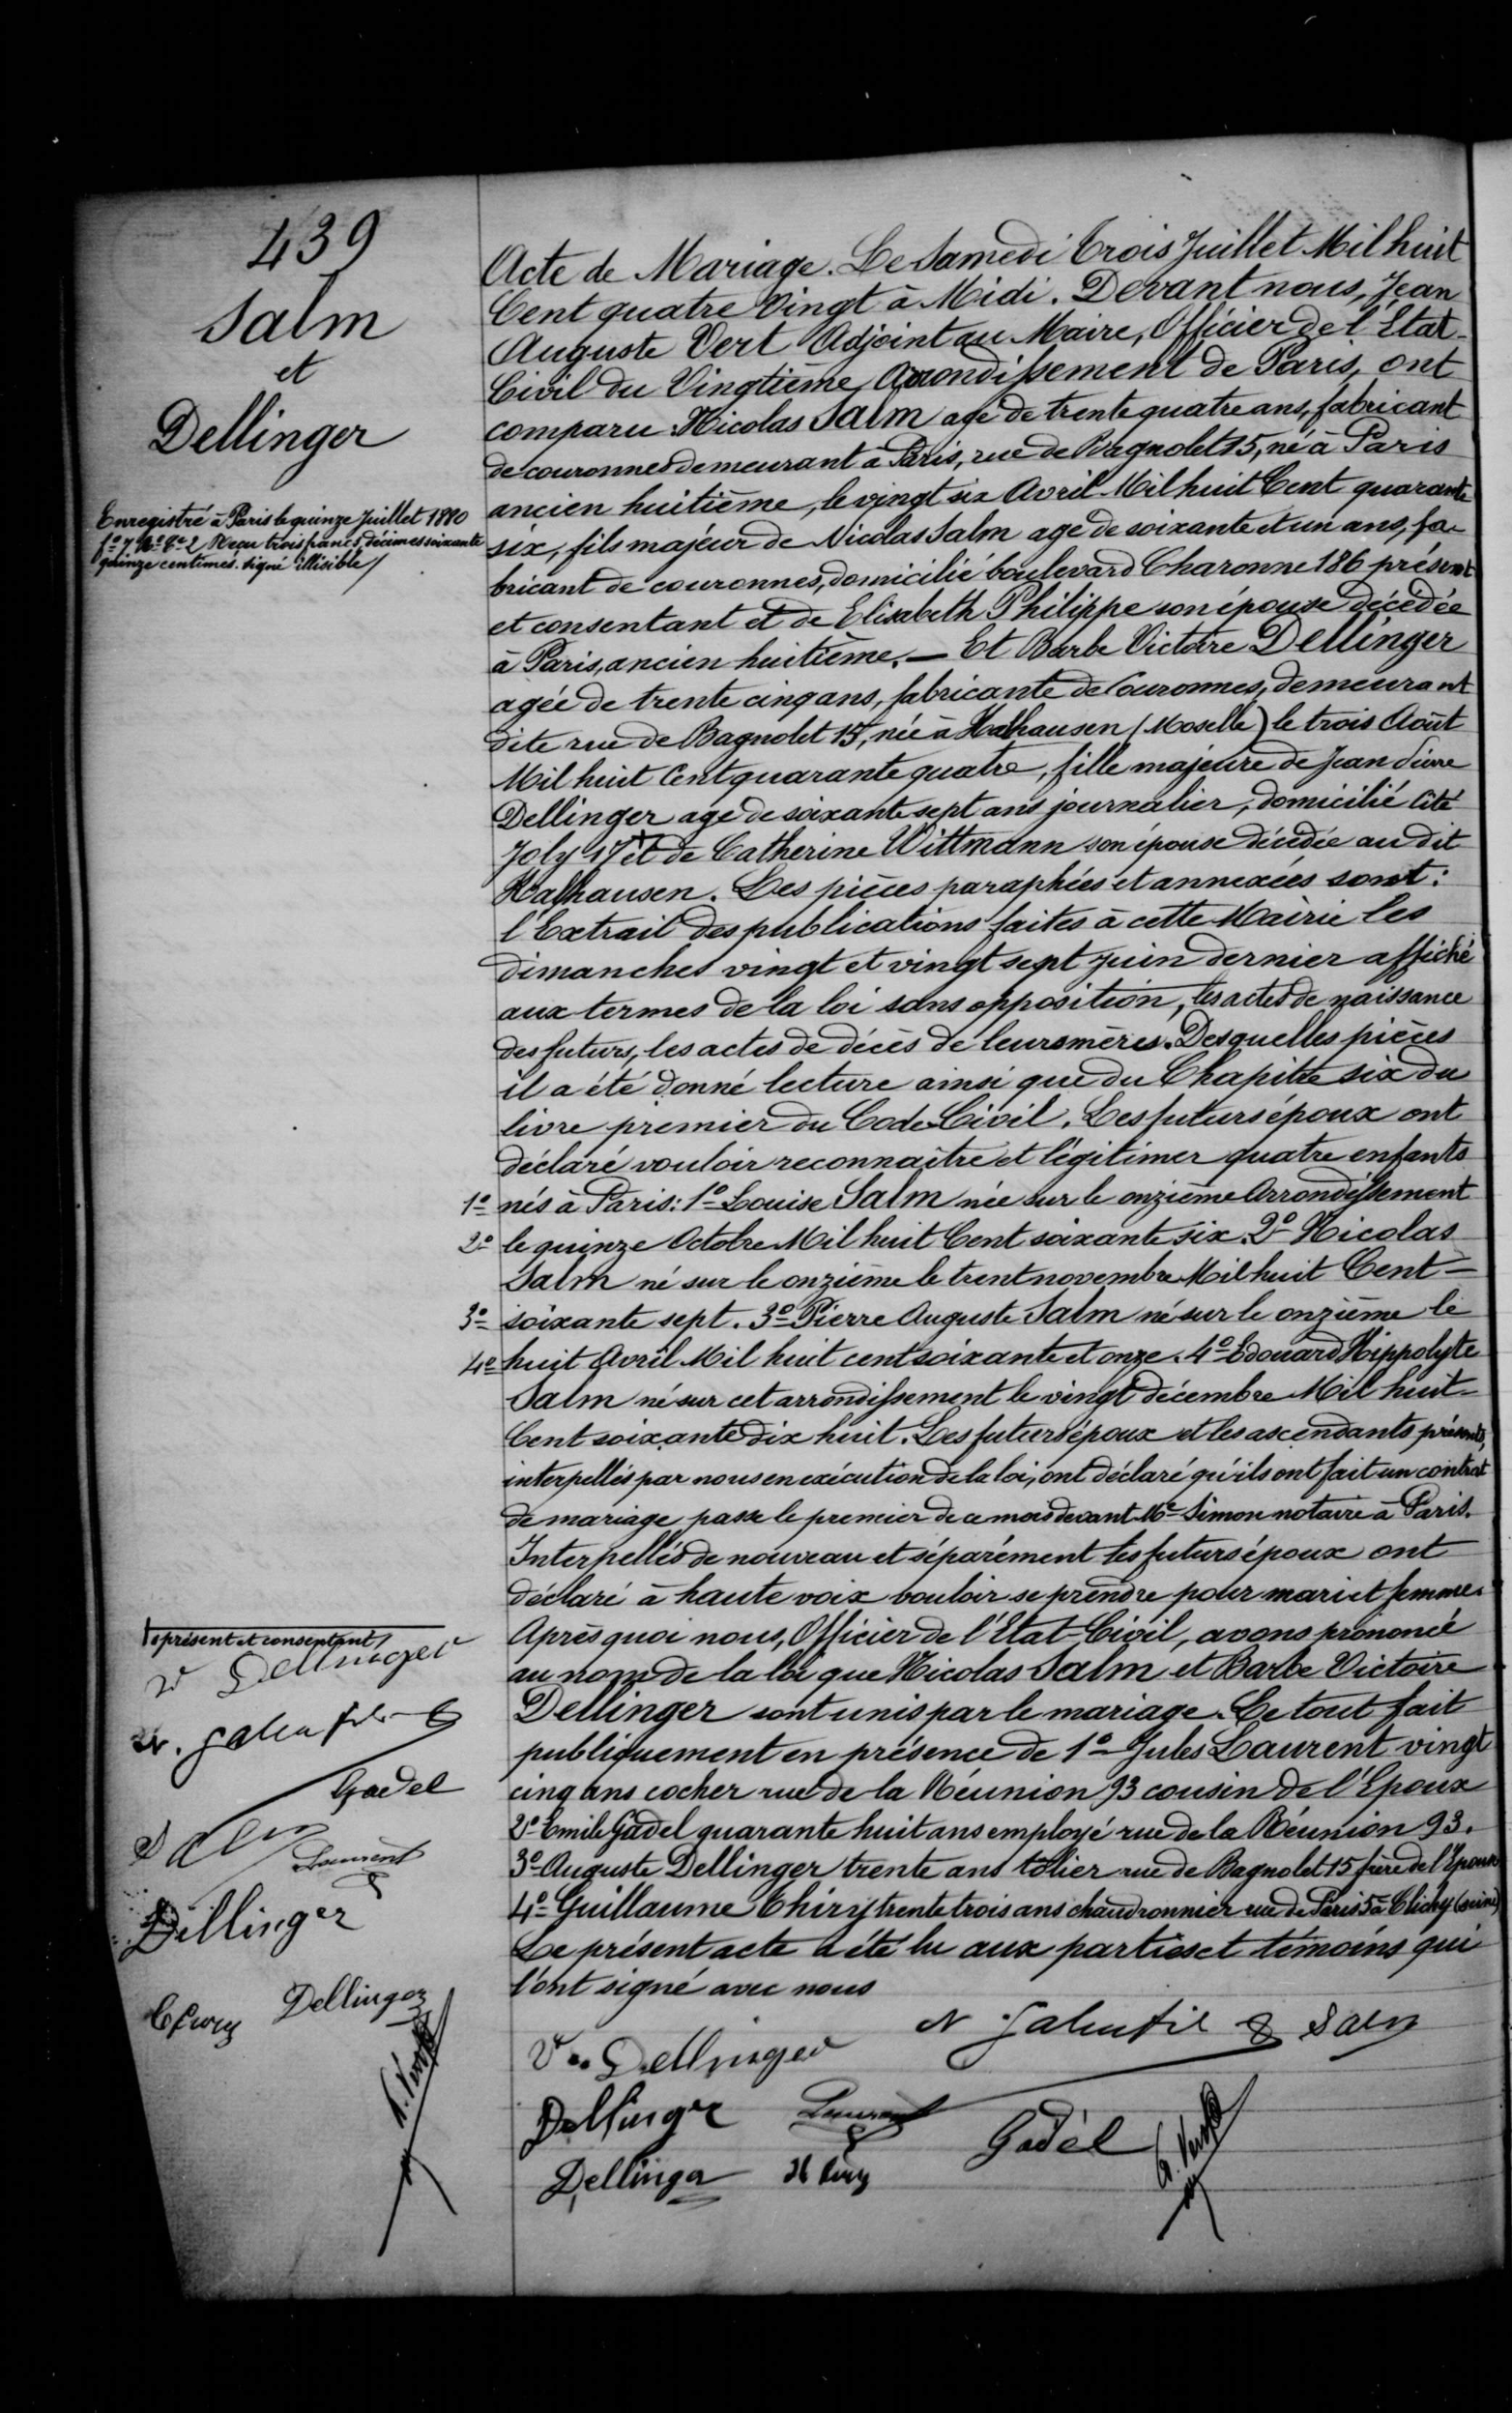439
Salm
et
Dellinger
Acte de Mariage. Le Samedi trois Juillet Mil huit
Cent quatre Vingt à Midi. Devant nous, Jean
Auguste Vert Adjoint au Maire, Officier de l'Etat
Civil du Vingtième Arrondissement de Paris, ont
comparu Nicolas Salm agé de trente quatre ans, fabricant
de couronne demeurant à Paris, rue de Bagnolet 15, né à Paris
ancien huitième, le vingt six Avril Mil huit Cent quarante
six, fils majeur de Nicolas Salm, age de soixante et un ans, fa
bricant de couronnes, domicilié boulevard Charonne 186 présent
et consentant et de Elisabeth Philippe son épouse décedée
à Paris, ancien huitième, -Et Barbe Victoire Dellinger
agée de trente cinq ans, fabricante de Couronnes, demeurant
dite rue de Bagnolet 15, née à Valhausen (Moselle) le trois Août
Mil huit cent quarante quatre, fille majeure de Jean Pierre
Dellinger, age de soixante sept ans journalier, domiciliée Cité
Joly 1 et de Catherine Wittmann son épouse décédée au dit
Kalhausen. Les pièces paraphées et annexées sont:
l'Extrait des publications faites à cette Mairie les
dimanches vingt et vingt sept Juin dernier affiché
aux termes de la loi sans opposition, les actes de naissance
des futurs, les actes de décés de leur mères. Desquelles pièces
il a été donné lecture ainsi que du Chapitre six du
livre premier du Code-Civil. Les futurs époux ont
déclaré vouloir reconnaître et légitimer quatre enfants
1° nés à Paris: 1° Louise Salm née sur le onzième Arrondissement
2° le quinze Octobre Mil huit Cent soixant-six. 2° Nicolas
Salm né sur le onzième le trente novembre Mil huit Cent-
3° soixante sept. 3° Pierre Auguste Salm né sur le onzième le
4° huit Avril Mil huit cent soixante et onze. 4° Edouard Hippolyte
Salm né sur cet arrondissement le vingt décembre Mil huit
Cent soixante dix huit. Les futurs époux et les ascendants présents
interpellés par nous en exécution de la loi, ont déclaré qu'ils ont fait un contrat
de mariage, passe le premier de ce mois devant M° Simon, notaire à Paris.
Interpellés de nouveau et séparément les futurs époux ont
déclaré à haute voix vouloir se prendre pour mari et femmes.
Après quoi nous, Officier de l'Etat-Civil, avons prononcé
au nom de la loi que Nicolas Salm et Barbe Victoire
Dellinger sont unis par le mariage. Le tout fait
publiquement en présence 1° Jules Laurent vingt
cinq ans cocher rue de la Réunion 93 cousin de l'Epoux
2° Emile Gadel quarante huit ans employé rue de la Réunion 93,
3° Auguste Dellinger trente ans tolier rue de Bagnolet 15 frère de l'Epouse
4° Guillaume Thiry trente trois ans chaudronnier rue de Paris 5 à Clichy (seine)
Le présent acte a été lu aux parties et témoins qui
l'ont signé avec nous
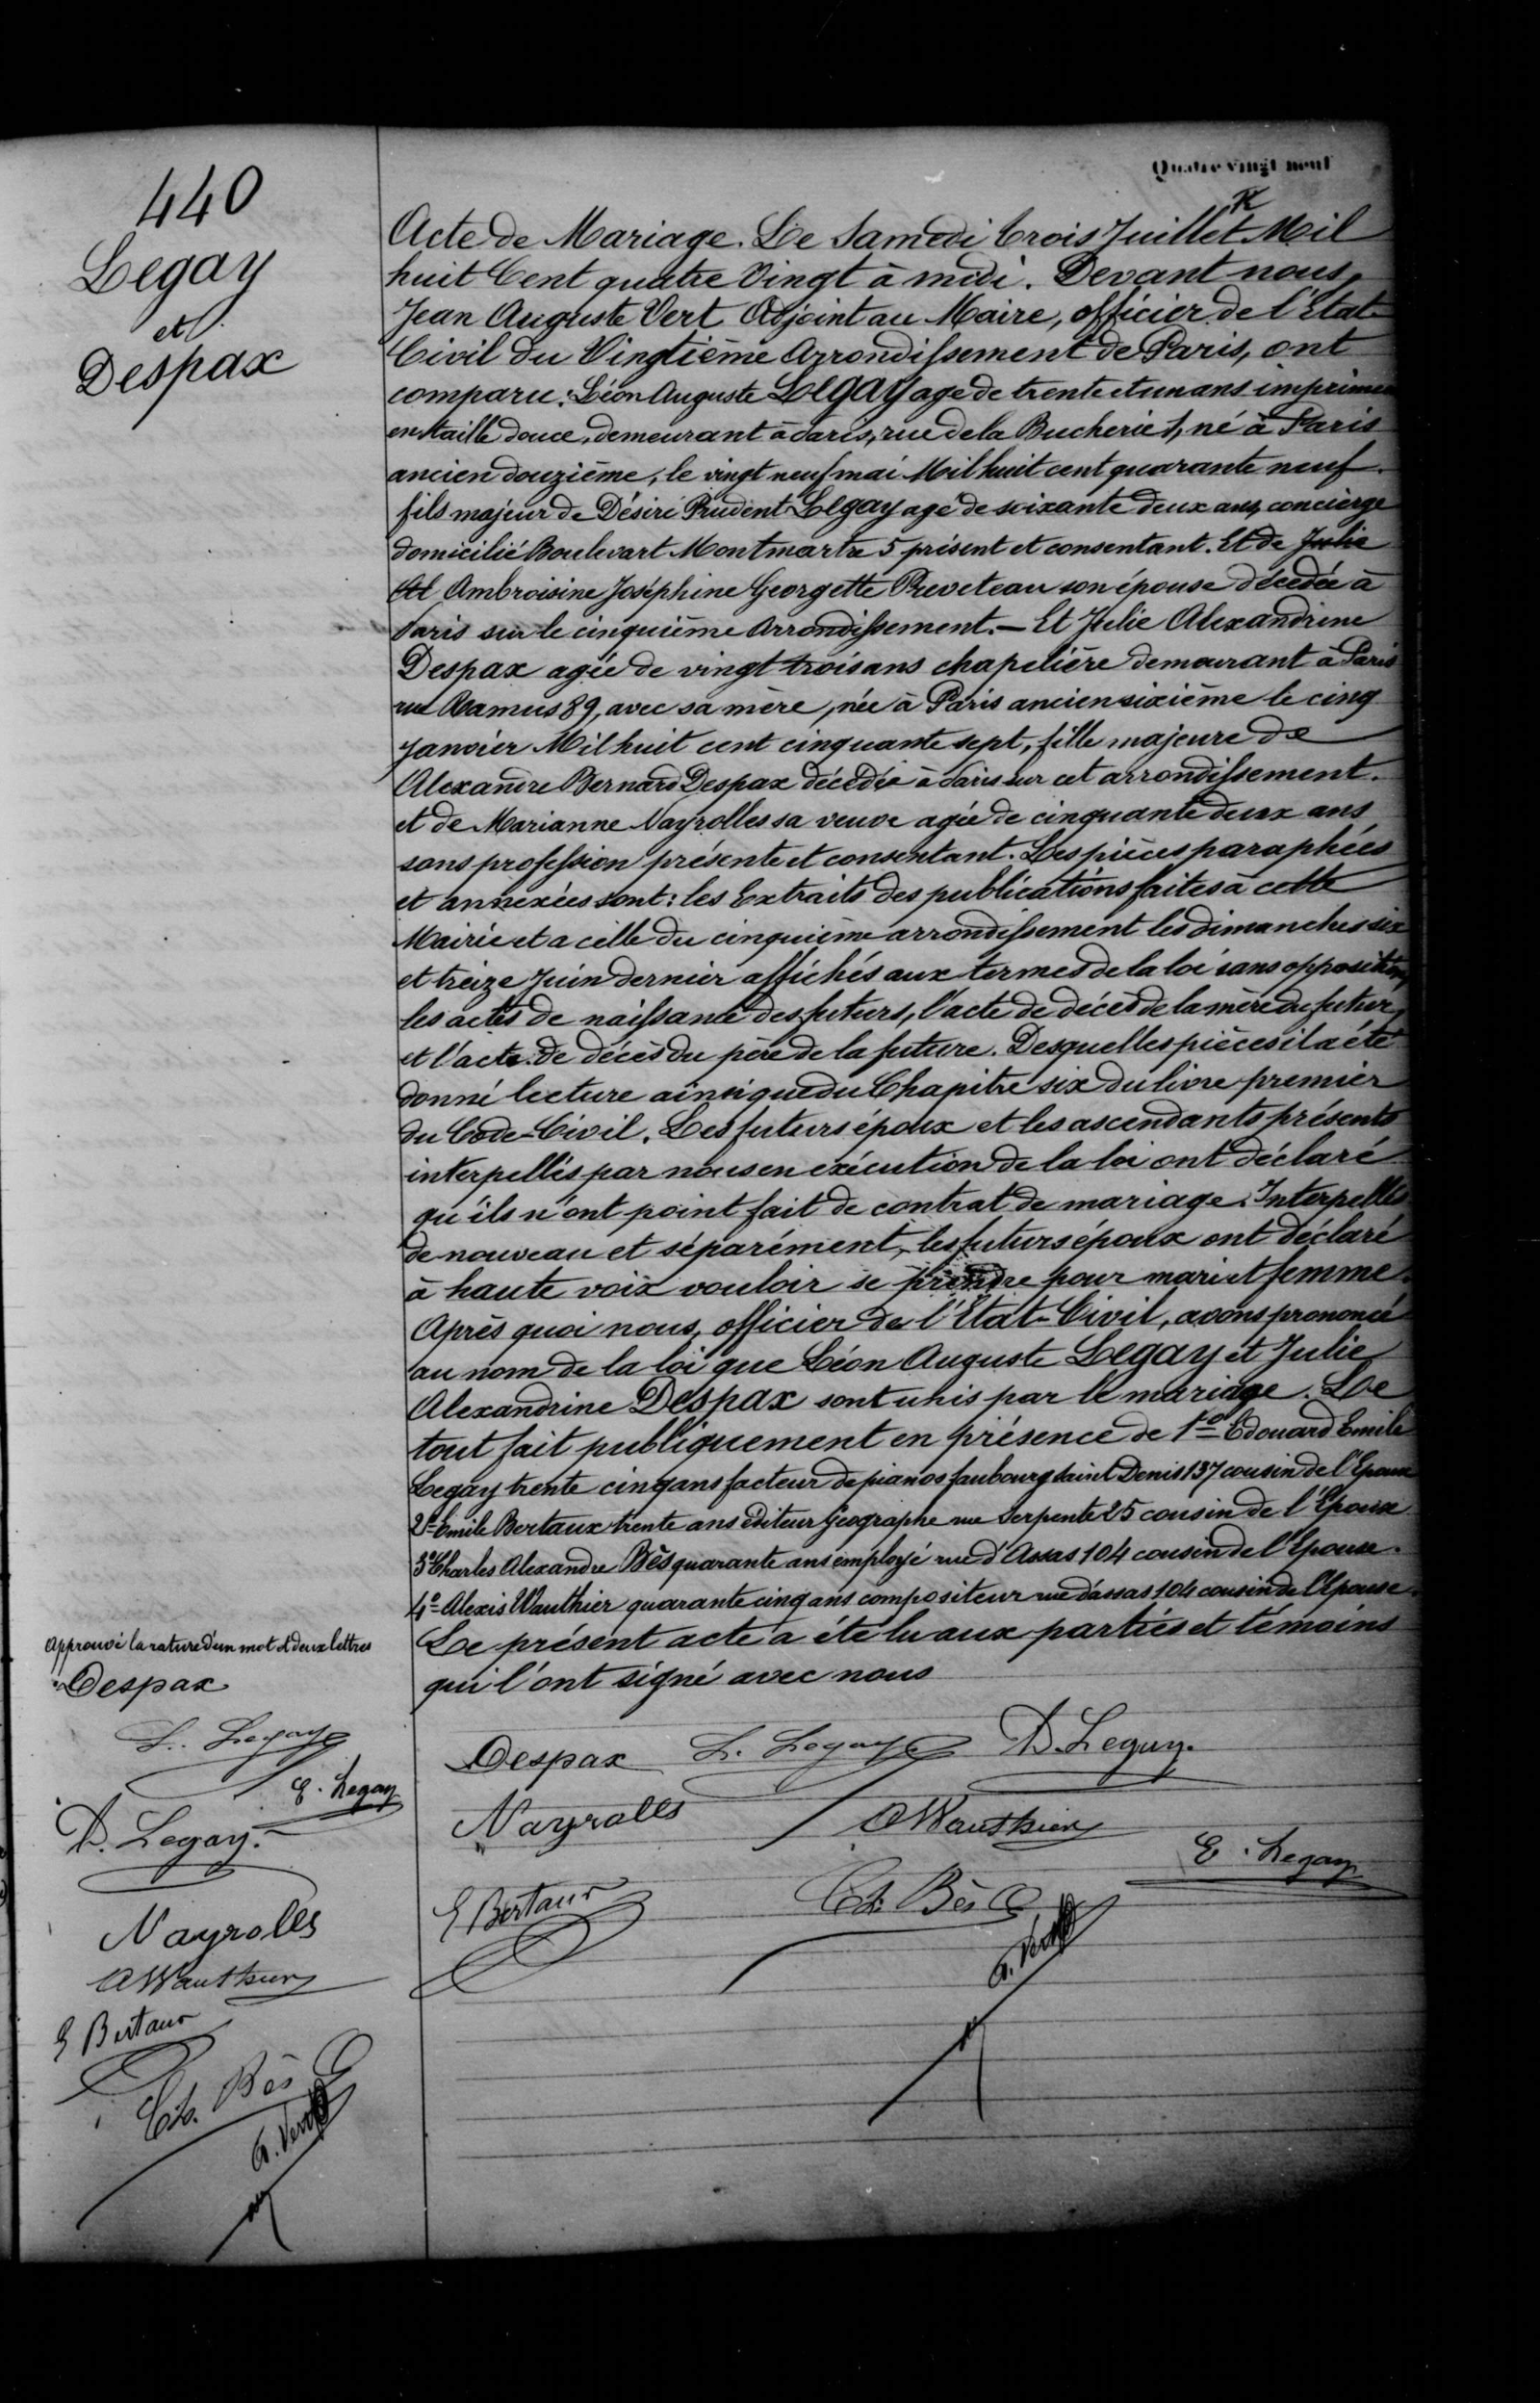440
Legay
et
Despax
Acte de Mariage. Le Samedi Trois Juillet Mil
huit Cent quatre Vingt à midi. Devant nous
Jean Auguste Vert Adjoint au Maire, officier de l'Etat-
Civil du Vingtième Arrondissement de Paris, ont
comparu. Léon Auguste Legay age de trente et un ans imprimeur
en taille douce, demeurant à Paris, rue de la Bucherie 1, né à Paris
ancien douzième, le vingt neuf mai Mil huit cent quarante neuf.
fils majeur de Désiré Rudent Legay agé de soixante deux ans concierge
domicilié Boulevard Montmartre 5 présent et concentant. Et de Julie
Ambroisine Joséphine Georgette Brevetau son épouse décédée à
Paris sur le cinquième Arrondissement.-Et Julie Alexandrine
Derpax agée de vingt trois ans chapelière demeurant à Paris
rue Namus 89, avec sa mère, née à Paris ancien sixième le cinq
Janvier Mil huit cent cinquante sept, fille majeure de
Alexandre Bernard Despax décédée à Paris sur cet arrondissement.
et de Marianne Nayrolles sa veuve agée de cinquante deux ans
sans profession présente et consentant. Les pièces paraphées
et annexées sont: les Extraits des publications faites à cette
Mairie et à celle du cinquième arrondissement les dimanches six
et treize Juin dernier affichés aux termes de la loi sans opposition
les actes de naissance des futurs, l'acte de décès de la mère du futur,
et l'acte de décès du père de la future. Desquelles pièces il a été
donné lecture ainsi que du Chapitre six du livre premier
du Code-Civil. Les futurs époux et les ascendants présents
interpellés par nous en exécution de la loi ont déclaré
qu'ils n'ont point fait de contrat de mariage. Interpellés
de nouveau et séparément, les futurs époux ont déclaré
à haute voix, vouloir se prendre pour mari et femme.
Après quoi nous officier de l'Etat-Civil, avons prononcé
au nom de la loi que Léon Auguste Legay et Julie
Alexandrine Despax sont unis pas le mariage. Le
tout fait publiquement en présence de 1° Edouard Emile
Legay trente cinq ans facteur de pianos faubourg Saint Denis 137 cousin de l'Epoux
2° Emile Bertraux trente ans éditeur Géographe rue Serpente 25 cousin de l'Epoux
3° Charles Alexandre Bès quarante ans employé rue d'Assas 104 cousin de l'Epouse.
4° Alexis Wauthier quarante cinq ans compositeur rue d'assas 104 cousin de l'Epouse
Le présent acte a été lu aux parties et témoins
qui l'ont signé avec nous